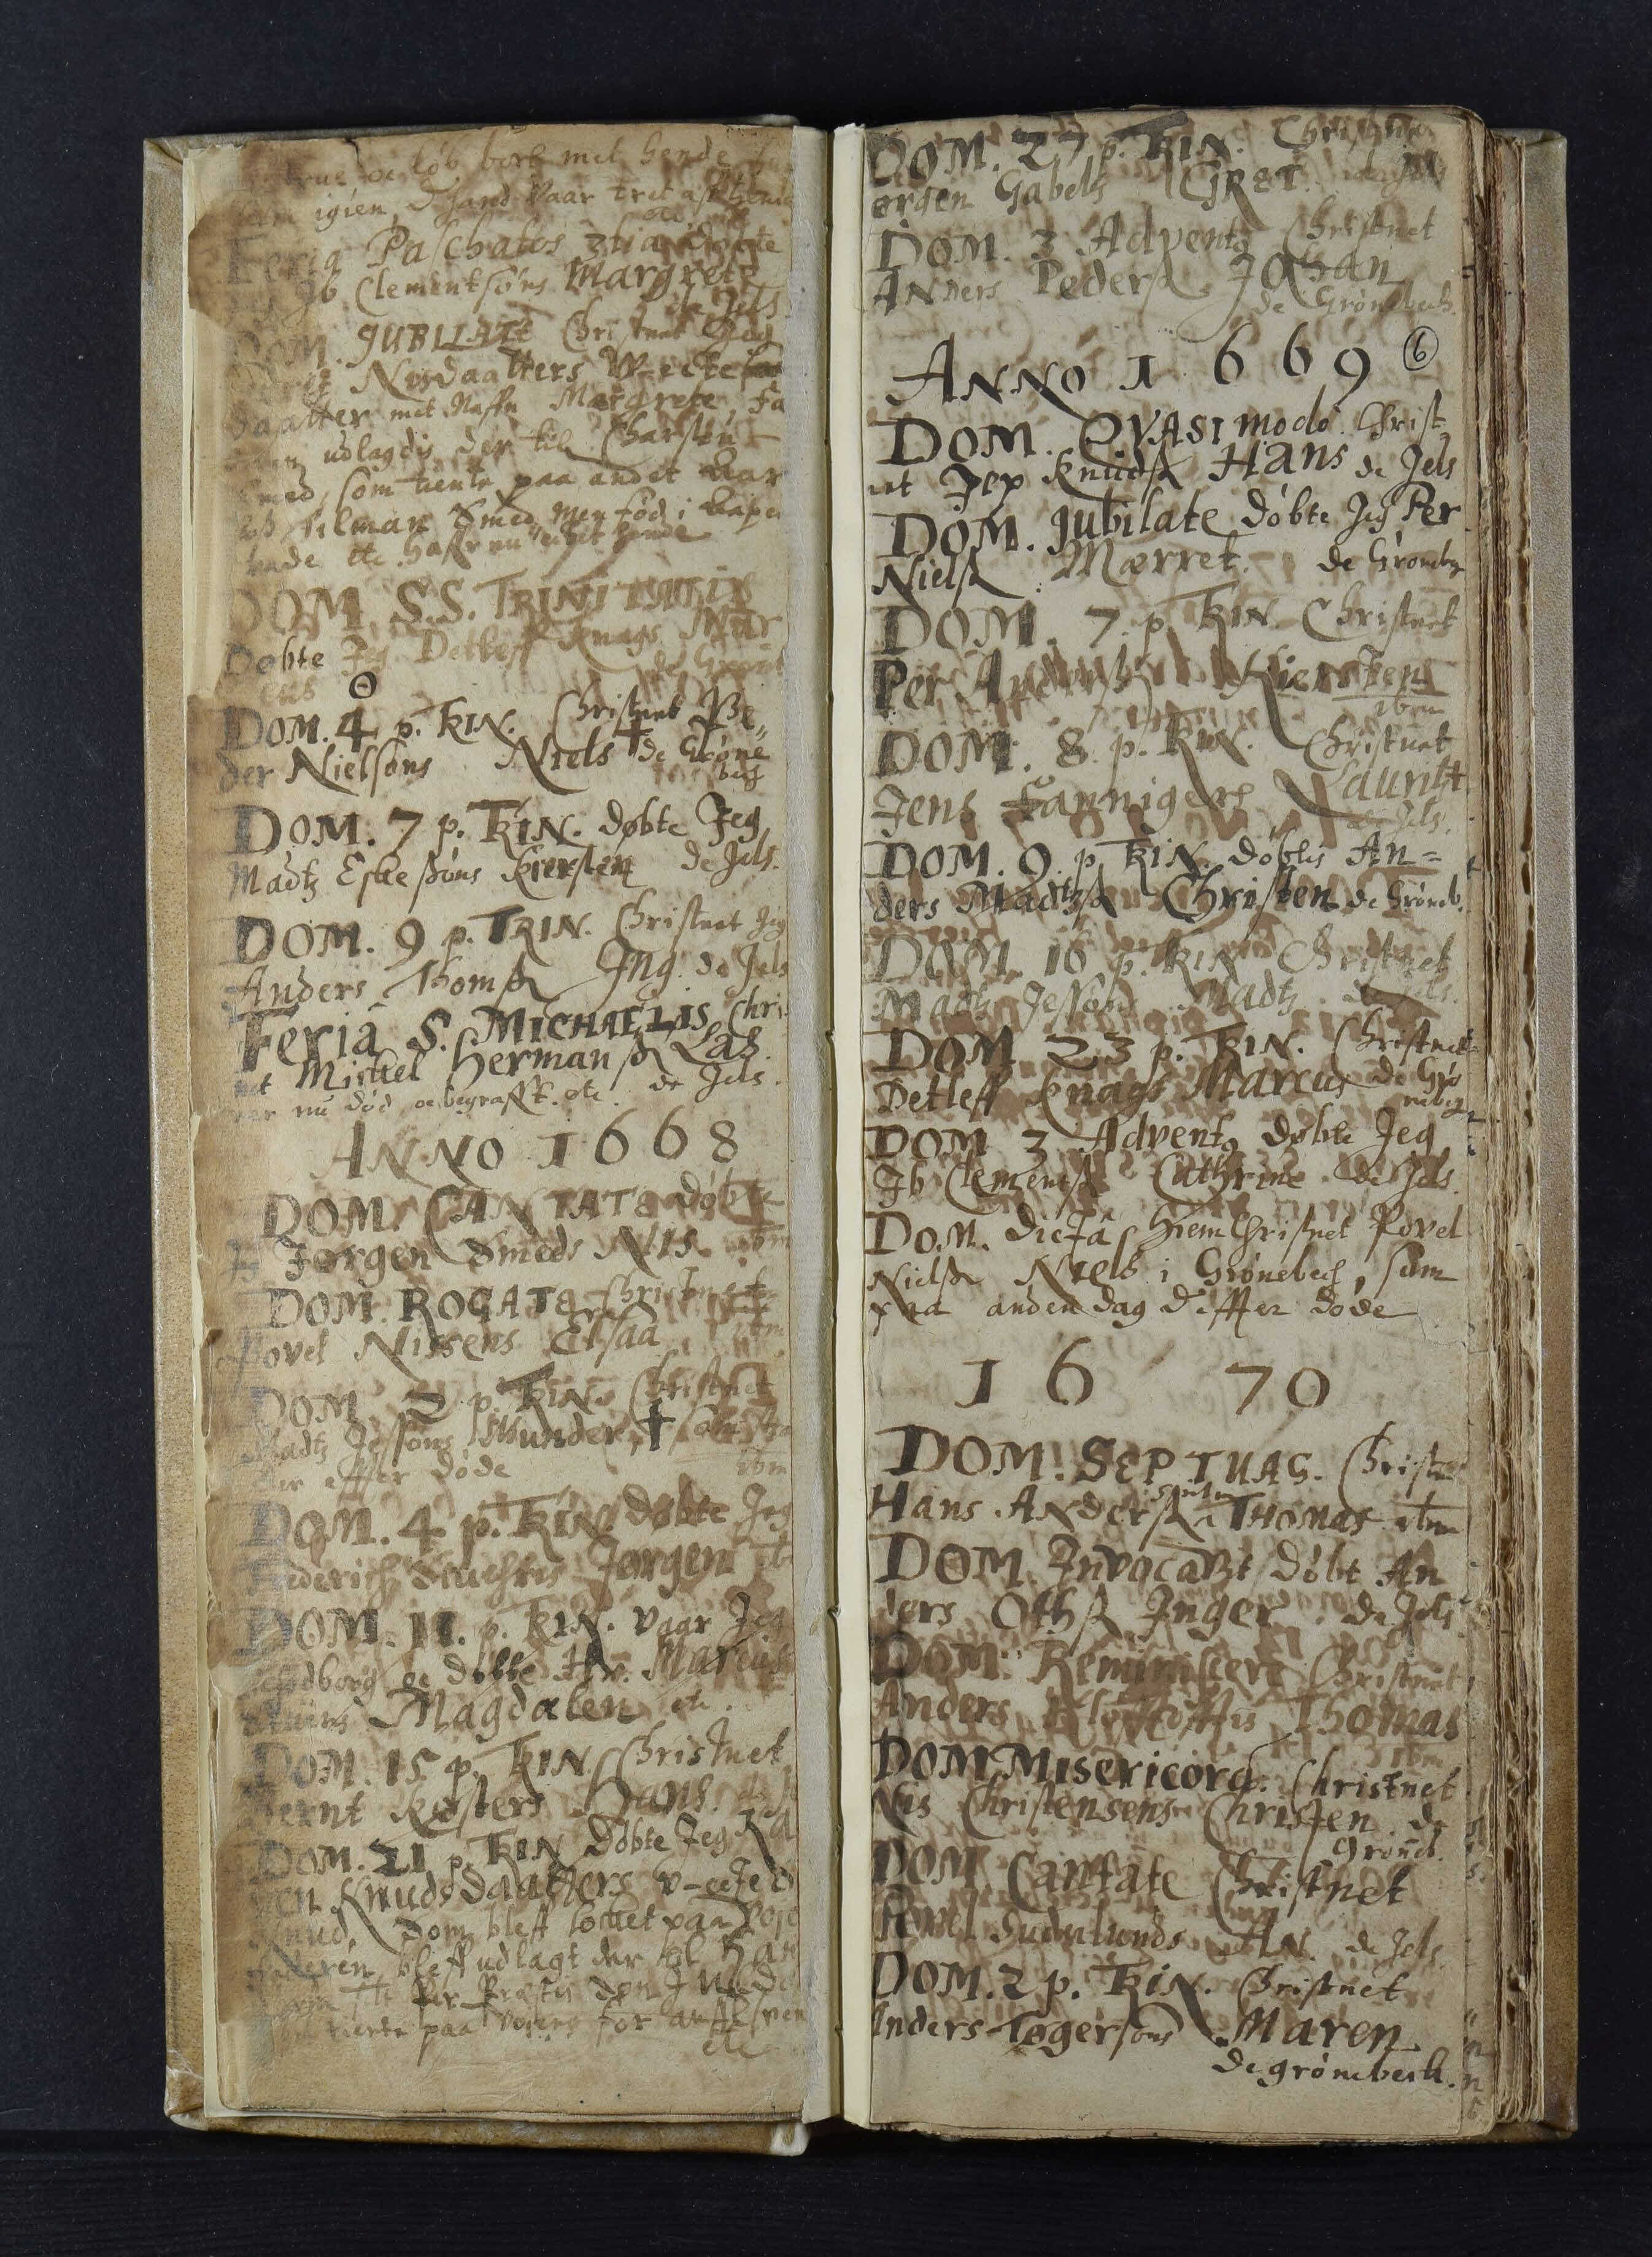##true oc løb bort med hende ##
igien, D hand Vaar tret aff hende
Feria Paschatos 3tia Døbte
Jeg Ib Clementsøns Margrete
de Jels.
DOM JUBILATE Christnet Jeg
##ret Nisdaatters V-ecte
Daatter met Naffn Margrete, Fa
deren## udlagdis der til Charsten
Smed, som tiente paa andet Aar
hos Tilman Smed men fød i Aapen
rade etc. Haffe nu ectet hende:
DOM. S. S. TRINITATIS
Døbte Jeg Detleff Knags Mar
cus Θ
de Grønb
Dom. 4 p. TRIN. Christnet Pe¬
der Nielsøns Niels de Grøne
bech
Dom. 7 p. TRIN. Døbte Jeg
Madtz Eskesøns Kiersten de Jels.
DOM. 9 p. TRIN. Christnet Jeg
Anders Thoms Ing de Jels
Feria S. MICHAELIS Chri¬
net Mickel Hermans Las.
nu død oc begrafft. etc. de Jels.
ANNO 1668
DOM. CANTATE Døbte
Jeg Jørgen Smeds NIS ibm
DOM. ROGATE Christnet
Povel Nissens Elsaa ibm
Dom. 2 p. Trin. Christnet
Madtz Jessøns Gunder, † som stra 
der efter døde ibm
ibm
DOM. 4 p. TRIN. Døbte Jeg
Frederich Stuchris Jørgen ib 
DOM. 11 p. TRIN. vaar Jeg
##chodborg oc døbte Hr. Marcus
Stuers Magdalen etc
DOM. 15 p. TRIN. Christnet
Bernt Køsters Hans de Je 
DOM. 21 p. TRIN. Døbte Jeg Ka
ren KnudsDaatters v-ecte
Knud, som bleff locket paa Voje
Faderen bleff udlagt der til Han 
Per Præstis den J
tiente paa Vojens for
etc
DOM. 27 p. TRIN. Christne
ørgen Gabels Gret de Jels
DOM 3 Adventq Christnet
Anders Peders Johan
de Grønebech.
ANNO 1669
DOM. QVASI modo Crist¬
net Jep Knuds Hans de Jels
DOM. Jubilate Døbte Jeg Per
Niels Mærret de Grøneb
DOM. 7 p. TRIN. Christnet
Per Anders## Kiersten
ibm
DOM 8 p. TRIN. Christnet
Jens Fannigers Lauritz
de Jels.
DOM 9 p. TRIN. Døbtes An¬
ders Madtzs Christen de Grøneb.
DOM. 16 p. TRIN Christnet
Madtz Jessøns Madtz. de Jels.
DOM 23 p. TRIN. Christnet
Detleff Knags Marcus de Grø
nebeg
DOM 3 Adventq døbte Jeg
Ib Clements Cathrine de Jels.
DOM. Dieta Hiem Christnet Povel
Niels Niels i Grønebech, som
paa anden dag d effter døde
1670
DOM. SEPTUAG. Christnet
Spilm
Hans Anders Thomas ibm
DOM. Invocavit Døbt An
ders Oths Inger. de Jels
DOM: Reminiscere Christnet
Anders Klottoftis Thomas
DOM Misericord. Christnet
Nis Christens Christen de
Grøneb.
DOM. Cantate Christnet
Povel Suderlunds An. de Jels.
Dom. 2 p. TRIN. Christnet
Anders Tøgersøns Maren
de grønebeck
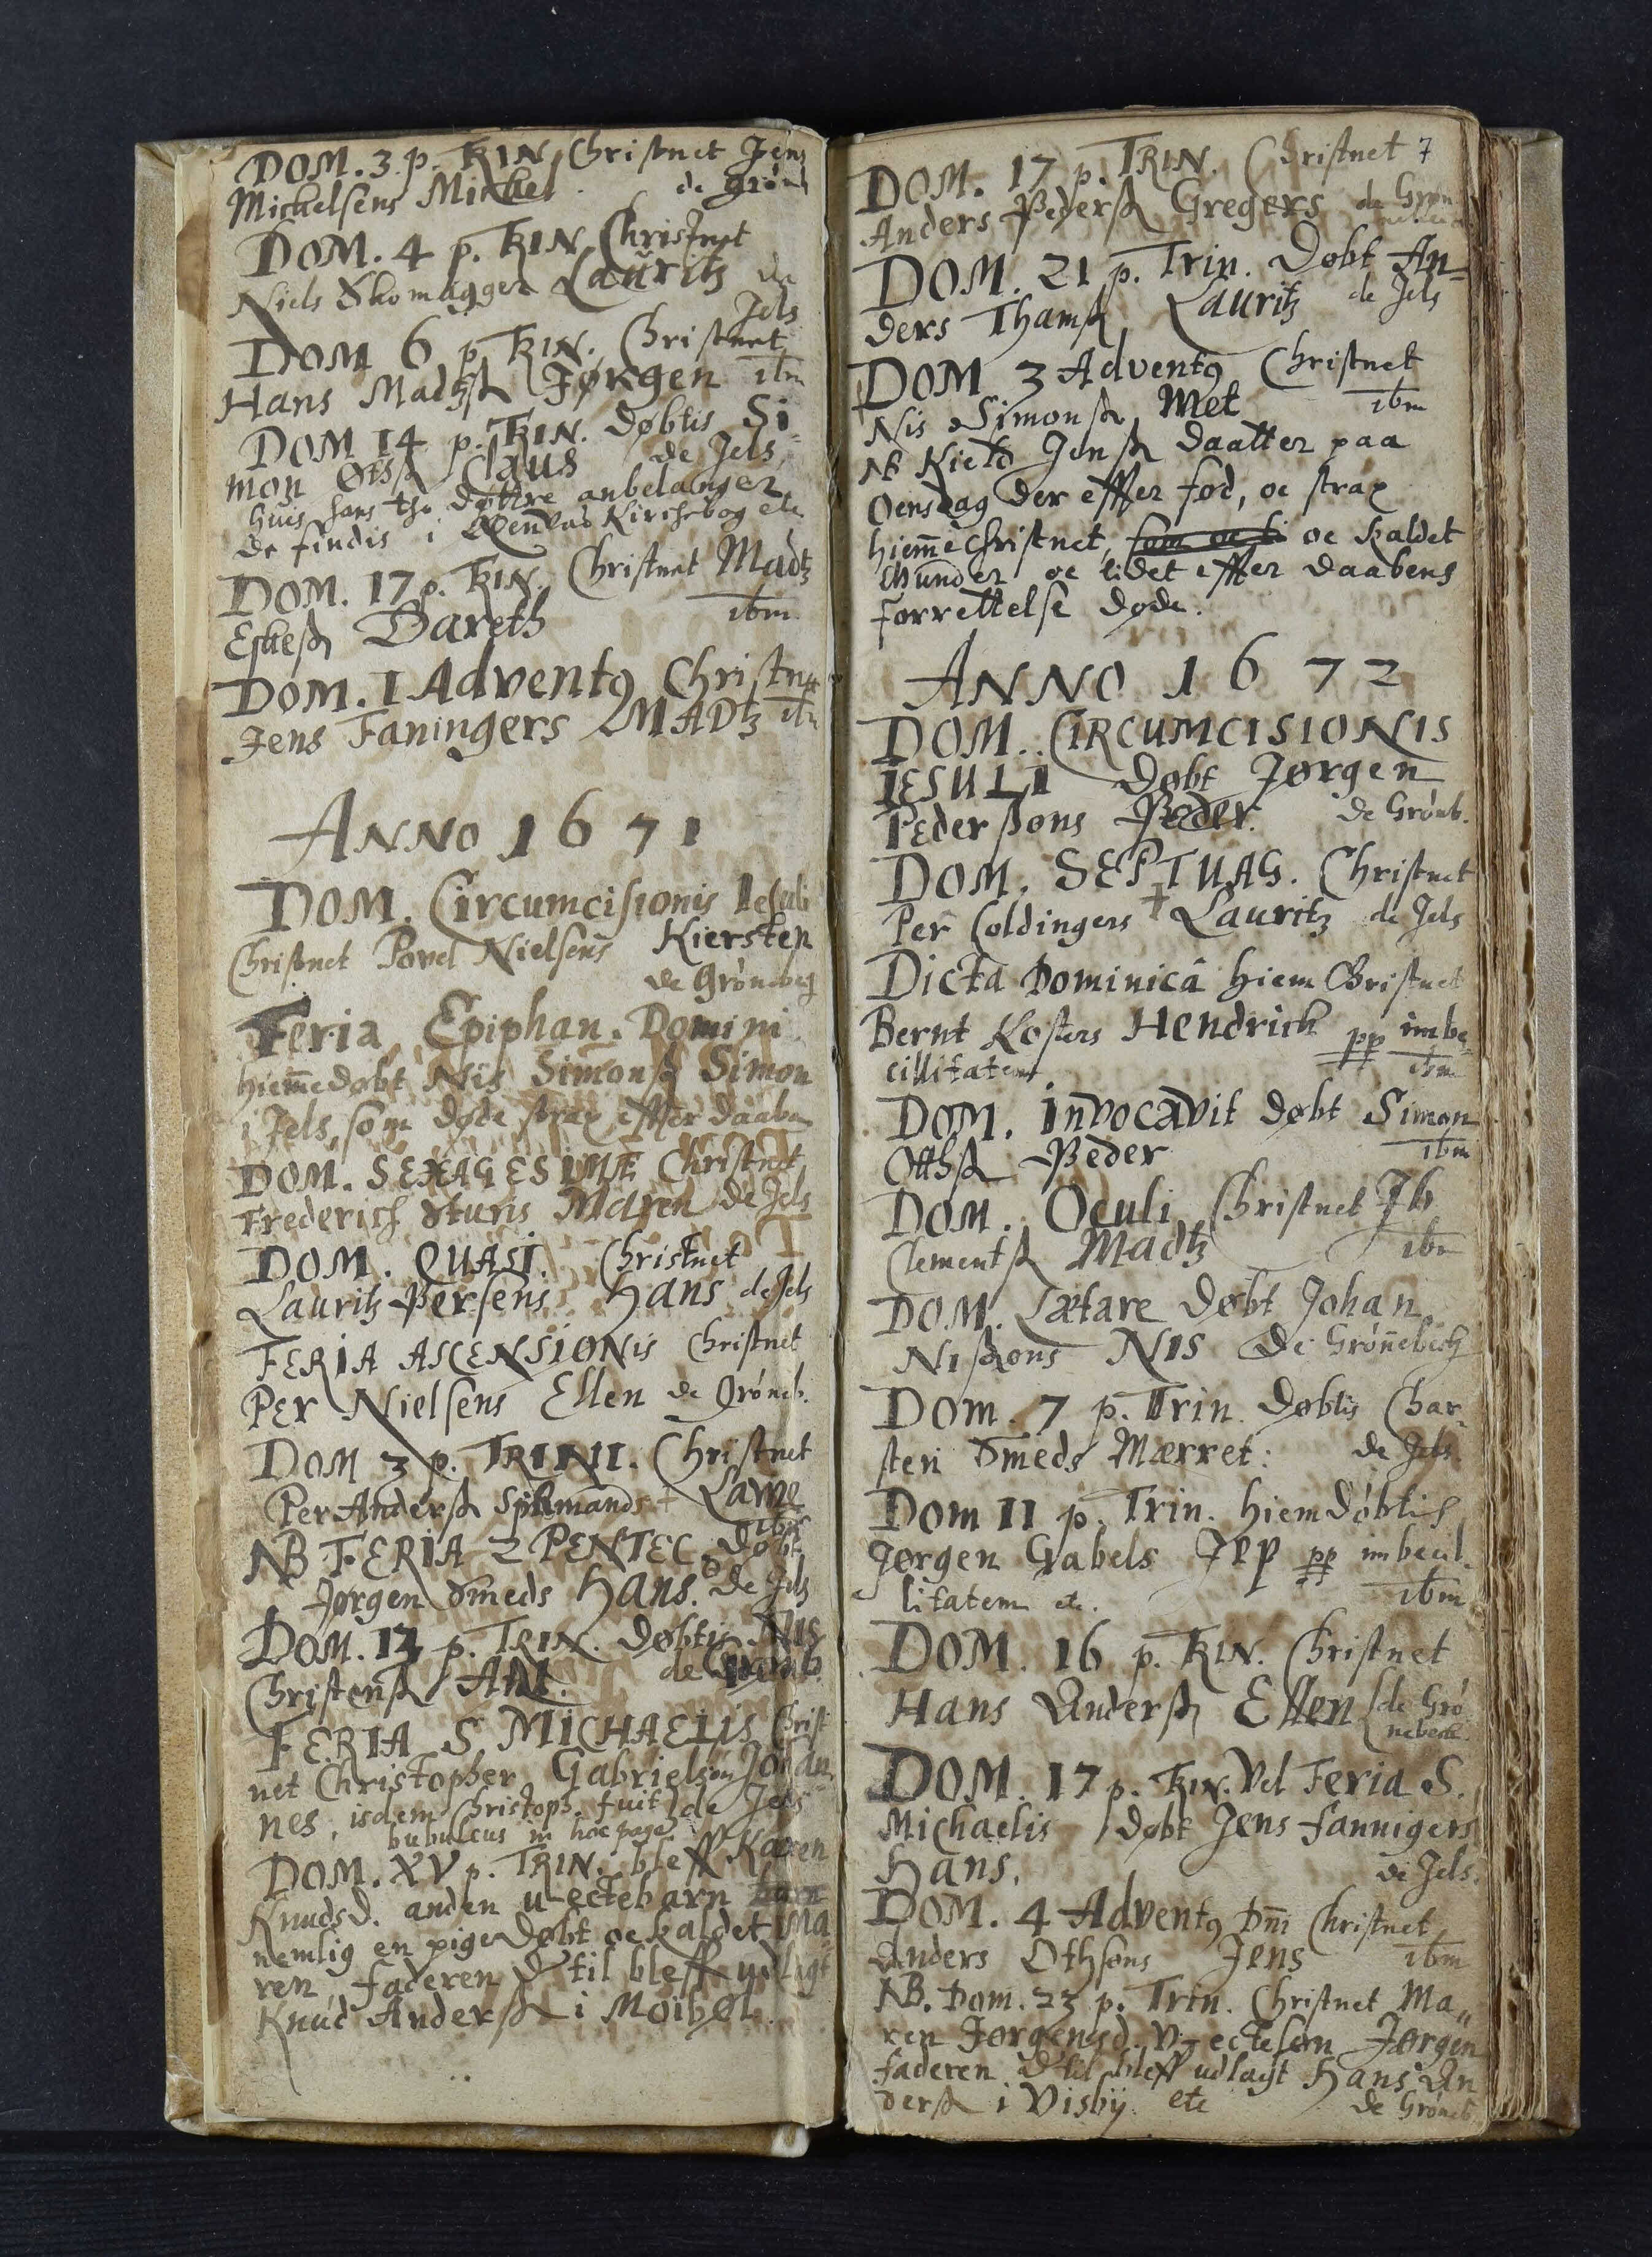DOM. 3. p. TRIN Christnet Jens
Mickelsens Mickel
de Grønb
DOM. 4 p. TRIN Christnet
Niels Skomagge## Laurits de
Jels
DOM 6 p. TRIN. Christnet
Hans Madtzs Jørgen ibm
DOM 14 p. TRIN. døbtis Si¬
mon Øtss Claus de Jels
huis hans tho Døttre anbelangen
de findis i Oxenvad Kirchebog etc.
DOM. 17 p. TRIN. Christnet Madtz
Eskes Dareth
ibm.
DOM. I Adventq Christnet
Jens Faningers MADTtz ibm
ANNO 1671
DOM. Circumcisionis Iesuli
Christnet Povel Nielsens Kiersten
de Grønebech
Feria Epiphan. Domini
Hiemmedøbt Nis Simons Simon
I Jels, som døde strax effter Daaben.
DOM. SEXAGESIMÆ Christnet
Frederich Sturis Maren de Jels
DOM. QUASI. Christnet
Lauritz Persens Hans de Jels
FERIA ASCENSIONis Christnet
Per Nielsens Ellen de Grøneb.
Dom 3 p. TRINIT. Christnet
Per Anders Spilmands + Lawe
ibm
NB FERIA 2 PENTEC Døbt
Θ
Jørgen Smeds Hans. de Jels
DOM. 13 p. TRIN. Døbtis NIS
Christens AN.
de Grøneb.
FERIA S MICHAELis Christ
net Christopher Gabrielsøn Johan¬
nes, iodem Christoph. fuit de Jels
bubuleus in hoc page
DOM. XV p. TRIN. bleff Karen
Knudsd. anden u-ectebarn barn
nemlig en pige Døbt oc kaldet Ma¬
ren, faderen d til bleff udlagt
Knud Anders i Moibøl.
7
DOM. 17 p. TRIN. Christnet
Anders Peders Gregers de Grøn
nebech
DOM. 21 p. TRIN. Døbt An¬
ders Thams Lauritz de Jels
DOM 3 Adventq Christnet
Nis Simons Met
ibm
NB Kield Jenss Daatter paa
Oensdag der effter fød, oc strax
Hiemmechristnet,
oc kaldet
Gunder oc lidet efter Daabens
forrettelse døde.
ANNO 1672
DOM.. CIRCUMCISIONIS
IESULI Døbt Jørgen
Pedersons Peder. de Grønb.
DOM. SEPTUAG. Christnet
†
Per Coldingers Lauritz de Jels
Dicta Dominica Hiem Christnet
Bernt Kosters Hendrick pp imbe¬
cillitatem
ibm
DOM. Invocavit Døbt Simon
Otths Peder
ibm
DOM. Oculi Christnet Ib
Clements Madtz
ibm
DOM. Lætare Døbt Johan
Nissens NIS De Grønebech
Dom. 7 p. Trin. Døbtis Char¬
sten Smeds Mærret: de Jels
Dom 11 p. Trin. Hiem Døbtis
Jørgen Gabels Jep pp imbecil¬
litatem etc.
ibm
DOM. 16 p. TRIN. Christnet
Hans Anders Ellen
de Grø
nebech
DOM. 17 p. TRIN. Vel Feria S.
Michaelis døbt Jens Fannigers
Hans.
de Jels.
DOM. 4 Adventq Dni Christnet
Anders Othsøns Jens ibm
NB. Dom 23 p. Trin. Christnet Ma¬
ren Jørgensd. V-echte søn Jørgen
Faderen d til bleff udlagt Hans An
ders i Visby etc
de Grøneb